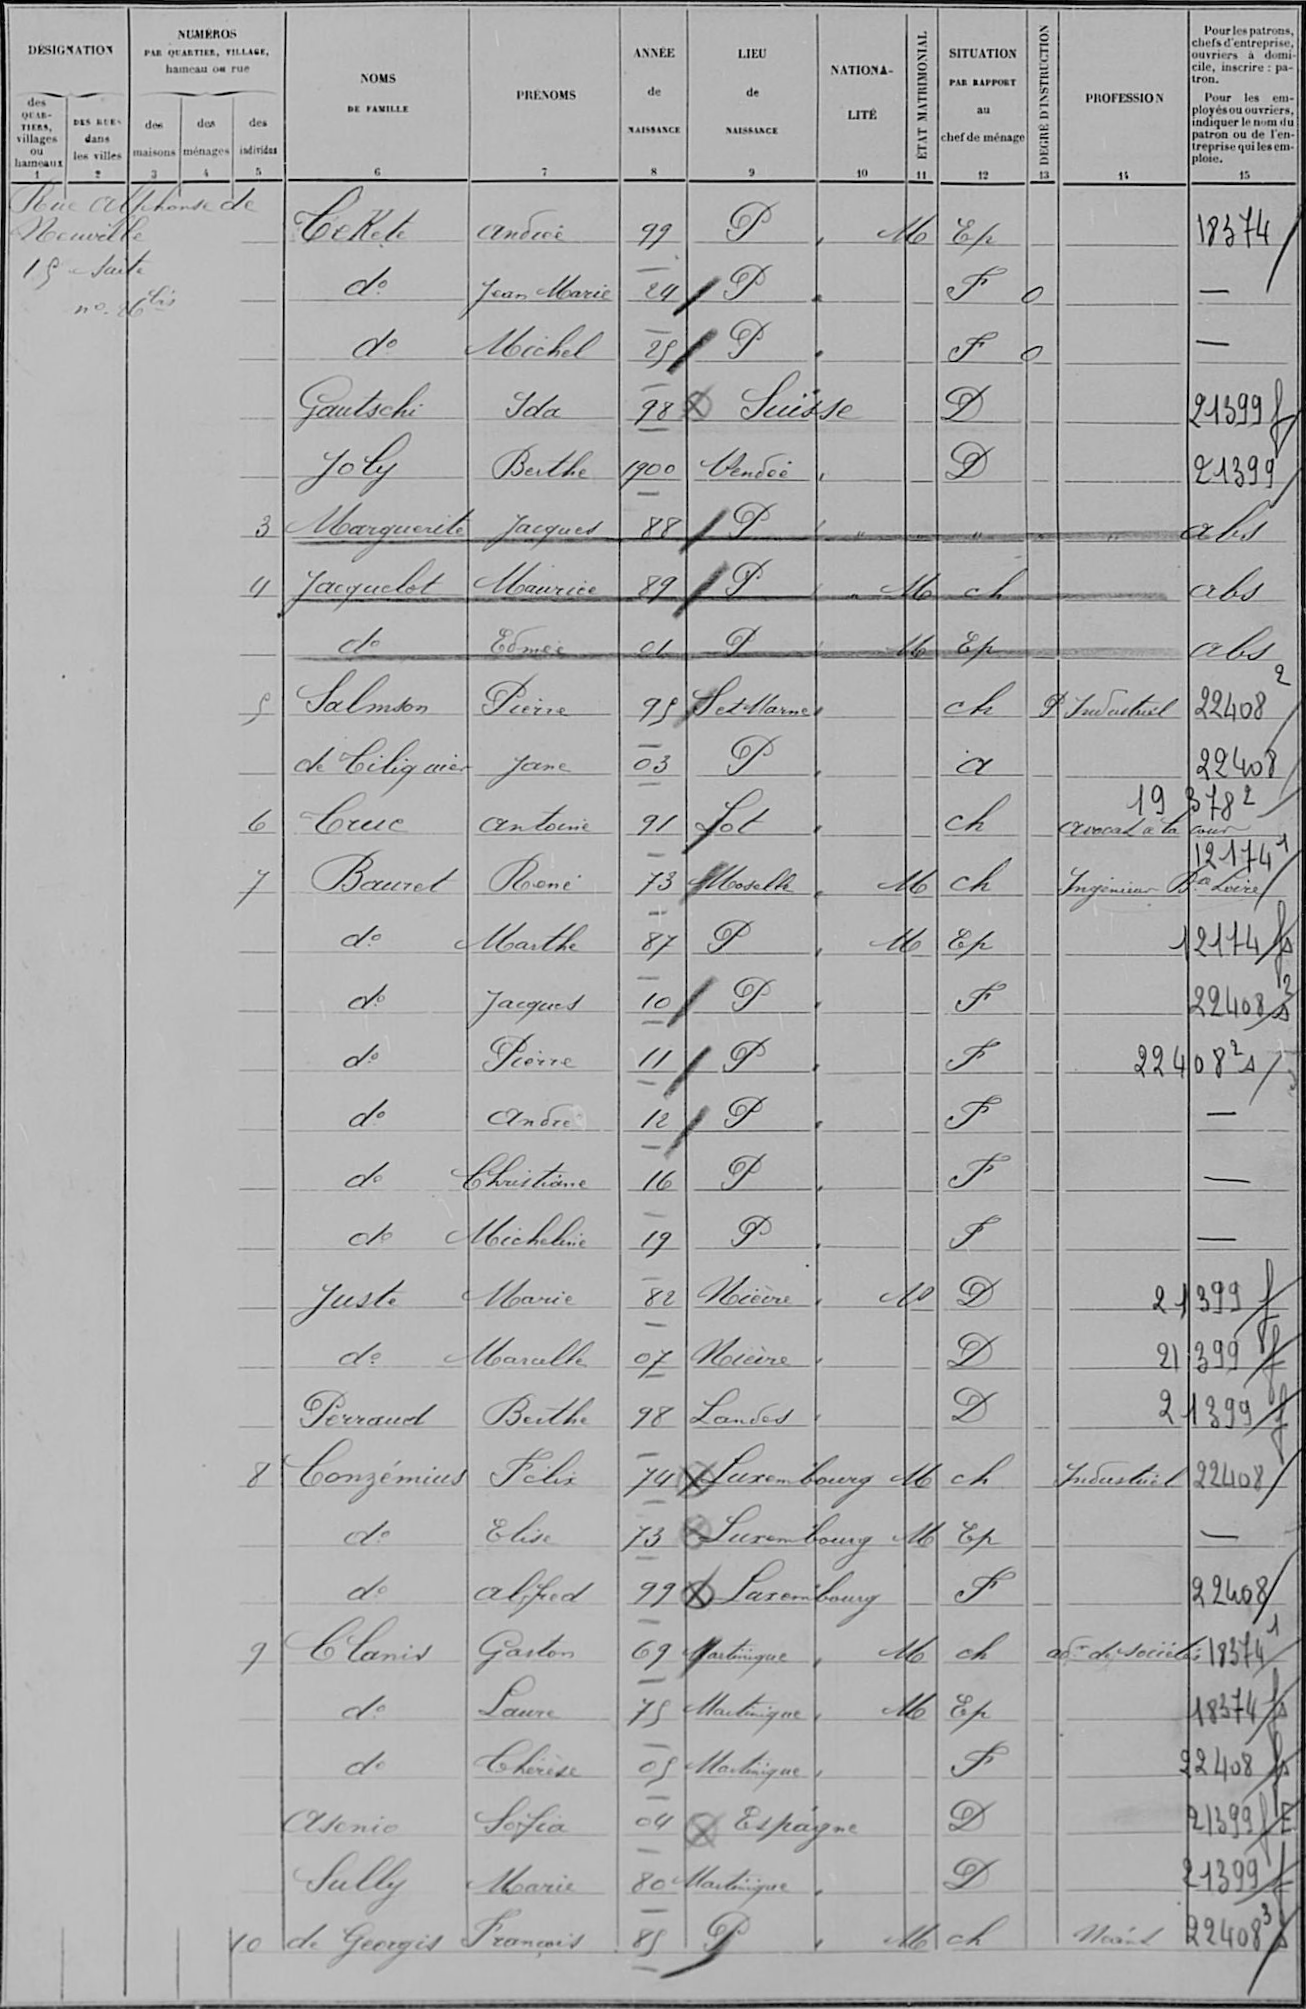Cekete/André/99/P/¤/M/Ep/¤/¤/18374
d°/Jean-Marie/24/P/¤/¤/F/o/¤/¤
d°/Michel/25/P/¤/¤/F/o/¤/¤
Gautschi/Ida/98/Suisse/¤/¤/D/¤/¤/21399
Joly/Berthe/1900/Vendée/¤/¤/D/¤/¤/21399
Marguerite/Jacques/88/P/¤/¤/¤/¤/¤/abs
Jacquelot/Maurice/89/P/¤/M/ch/¤/¤/abs
d°/Edmée/01/P/¤/M/Ep/¤/¤/abs2
Salmson/Pierre/95/S et Marne/¤/¤/ch/P/Industriel/224082
de Ciliguier/Jane/03/P/¤/¤/a/¤/¤/22408
Crue/Antoine/91/Lot/¤/¤/ch/¤/avocat à la cour/!193782
Bauret/René/73/Moselle/¤/M/ch/¤/Ingénieur Ba Loire/121741
d°/Marthe/87/P/¤/M/Ep/¤/¤/12174
d°/Jacques/10/P/¤/¤/F/¤/¤/22408
d°/Pierre/11/P/¤/¤/F/¤/¤/22408
d°/André/12/P/¤/¤/F/¤/¤/¤
d°/Christiane/16/P/¤/¤/F/¤/¤/¤
d°/Micheline/19/P/¤/¤/F/¤/¤/¤
Juste/Marie/82/Nièvre/¤/M/D/¤/¤/21399 f
d°/Marcelle/07/Nièvre/¤/¤/D/¤/¤/21399 f
Perraud/Berthe/98/Landes/¤/¤/D/¤/¤/21399 f
Conzémius/Félix/74/Luxembourg/¤/M/ch/¤/Industriel/22408
d°/Elise/73/Luxembourg/¤/M/Ep/¤/¤/¤
d°/Alfred/99/Luxembourg/¤/¤/F/¤/¤/22408
Clanis/Gaston/69/Martinique/¤/M/ch/¤/ad de société/183741
d°/Laure/75/Martinique/¤/M/ch/¤/¤/18374 fs
d°/Thérèse/05/Martinique/¤/¤/F/¤/¤/22408 fs
Asenio/Sofia/04/Espagne/¤/¤/D/¤/¤/21399fE
Sully/Marie/80/martinique/¤/¤/D/¤/¤/21399 f
de Georges/François/83/P/¤/M/ch/¤/Néant/224082
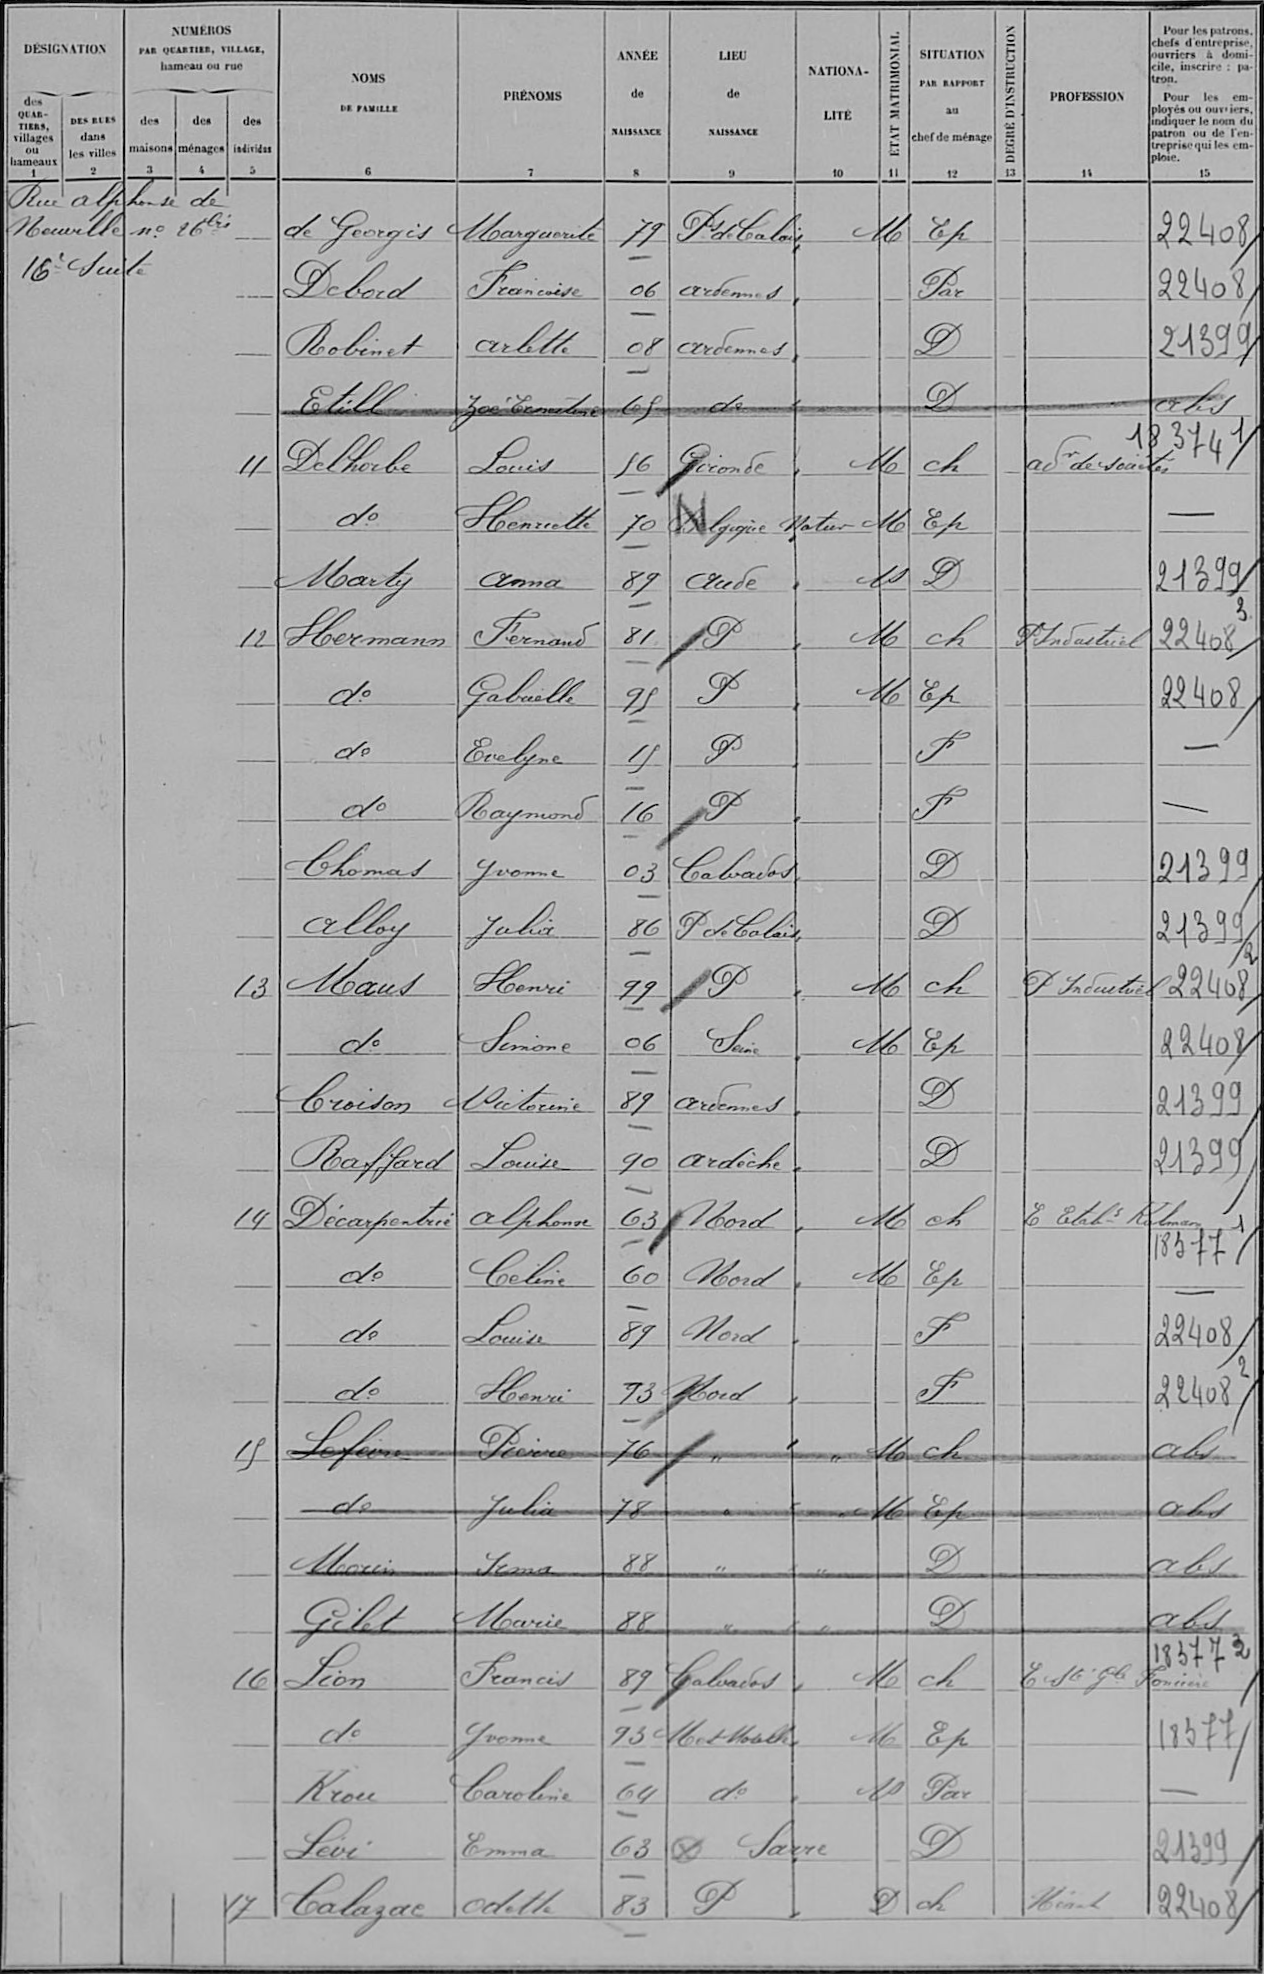de Georgis/Marguerite/79/P de Calais/¤/M/Ep/¤/¤/22408
Debord/Francoise/06/ardennes/¤/¤/par/¤/¤/22308
Robinet/Arlette/08/ardennes/¤/¤/D/¤/¤/21399
Etill/Zoé Ernestine/69/d°/¤/¤/D/¤/¤/abs1
Delhorbe/Louis/56/Gironde/¤/M/ch/¤/asr de sociétés/18374
d°/Henriette/70/Belgique/natur/M/Ep/¤/¤/¤
Marty/Anna/89/Aude/¤/M/D/¤/¤/21399
Hermann/Fernand/81/P/¤/M/ch/¤/P Industriel/224083
d°/Gabrielle/95/P/¤/M/Ep/¤/¤/22408
d°/Evelyne/19/P/¤/¤/F/¤/¤/¤
d°/Raymond/16/P/¤/¤/F/¤/¤/¤
Thomas/Yvonne/03/Calvados/¤/¤/D/¤/¤/21399
Alloy/Julia/86/P de Calais/¤/¤/D/¤/¤/213992
Maus/Henri/99/P/¤/M/ch/¤/P Industriel/22408
d°/Simone/06/Seine/¤/M/Ep/¤/¤/22408
Croison/Victorine/89/ardennes/¤/¤/D/¤/¤/21399
Raffard/Louise/90/ardèche/¤/¤/D/¤/¤/21399
Décarpentrie/Alphonse/63/Nord/¤/M/ch/¤/E etabs Kolman/!183771
d°/Celine/60/Nord/¤/M/Ep/¤/¤/18377
d°/Louise/89/Nord/¤/¤/F/¤/¤/22408
d°/Henri/93/Nord/¤/¤/F/¤/¤/224082
Sofeire/Pierre/76/¤/¤/M/ch/¤/¤/abs
d°/Julia/78/"/"/M/Ep/¤/¤/abs
Morin/Irma/88/¤/¤/¤/D/¤/¤/abs
Gilet/Marie/88/¤/¤/¤/D/¤/¤/abs
Léon/Francis/89/Calvados/¤/M/ch/¤/E Se gle Foncière/183772
d°/Yvonne/93/M et Moselle/¤/M/Ep/¤/¤/18377
Krou/Caroline/64/d°/¤/M/Par/¤/¤/¤
Lévi/Emma/63/Sarre/¤/¤/D/¤/¤/21399
Calazac/Odette/83/P/¤/D/ch/¤/Néant/22408